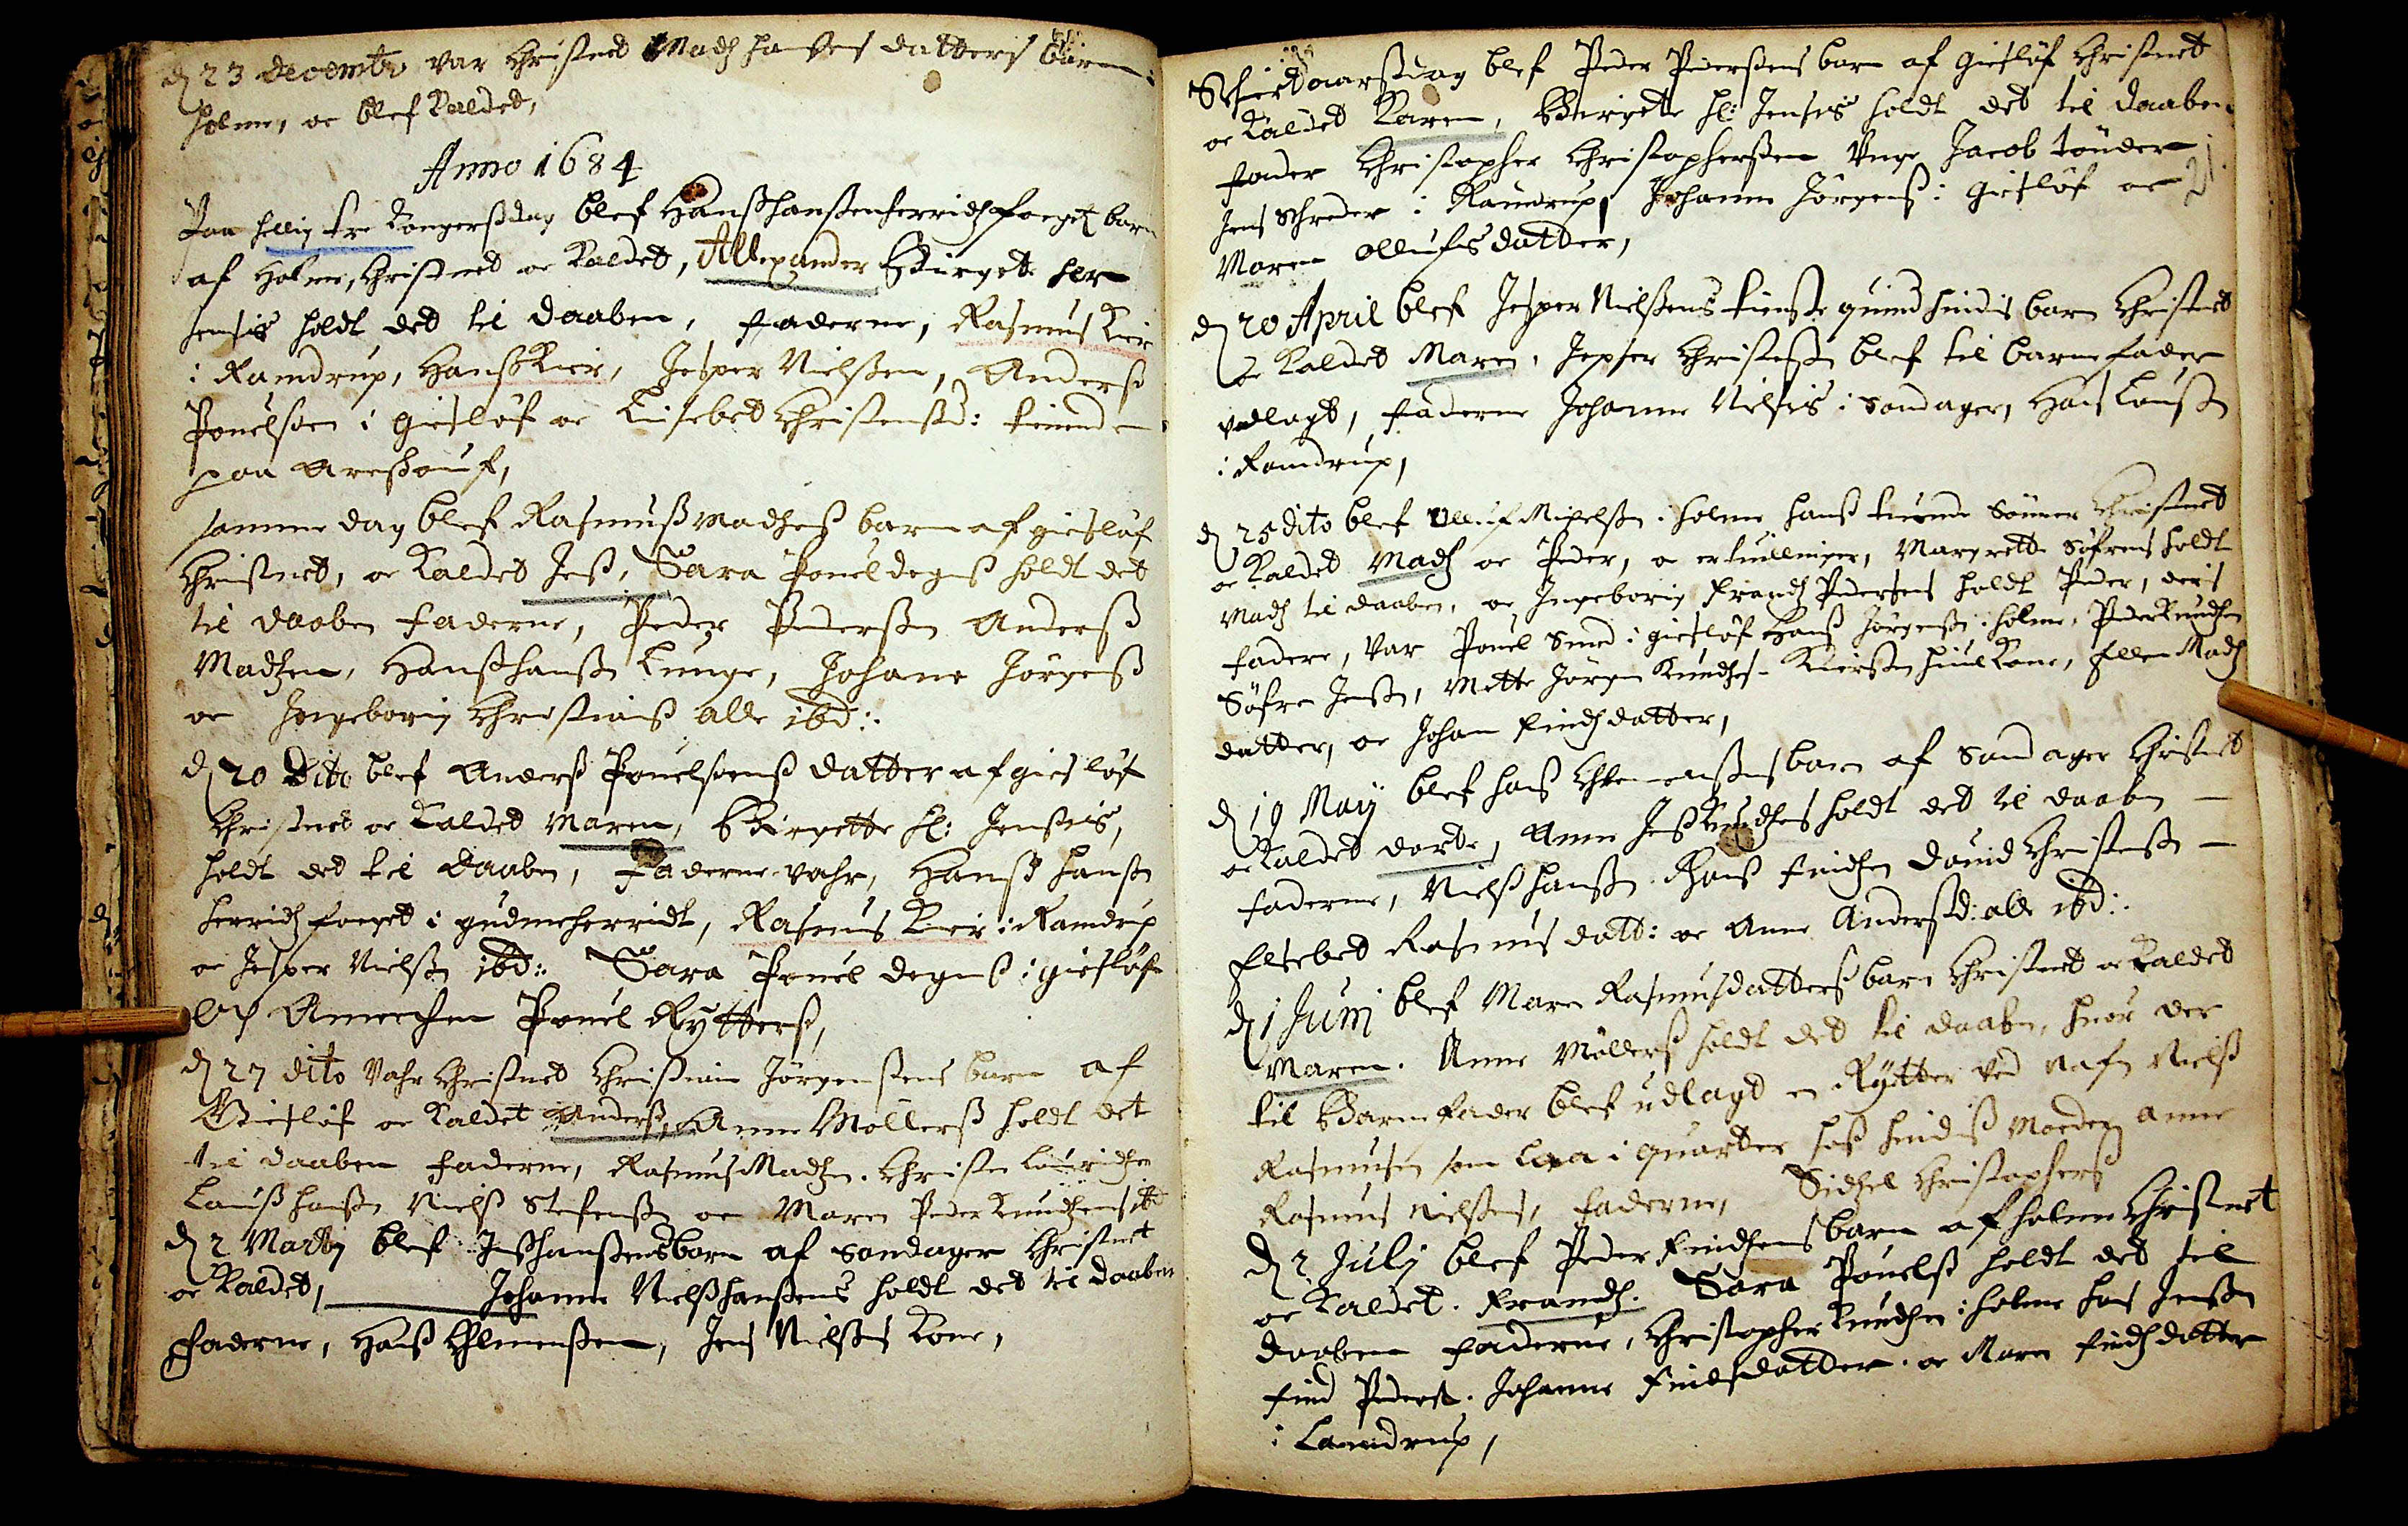d 23 Decembr var Christet Madtz Hansens datters barn i
Holme, oc blef kaldet,
Anno 1684
Paa hellig tre kongerssdag bleff Hanss Hanssen herridtzfoegets barn
af Holme Christened oc kaldet, Allexander Birgette hlr
Jensis holdt det til Daaben, Faderne; Rasmus Kier
i Ramdrup, Hans Kier, Jesper Nielssen, Anderss
Pouelsen i Gieløf oc Lisebet Christenssd: tiende
paa Areskouf,.
Samme dag blef Rasmuss Madtzens børn af giesløf
Christnet, oc kaldet Jens, Sara Pouel degnss holdt det
til daaben Faderne, Peder Pederssen Anderss
Madtzen, Hans Hanss Lunge, Johane Jørgenss
oc Ingeborig Christenss alle ibd:.
d 20 Dito blef Anderss Pouelsenss Datter af giesløf
Christnet oc kaldet Maren, Birrette Hl: Jenssis
holdt det til Daaben, Faderne vahr, Hanss Hanss
Herridtzfoeget i Gudmeherridt, Rasmus Kier i Ramdrup
oc Jesper Nielss ibd:. Sara Pouel Degns i giesløf
Och Anneche Pouel Rytterss,
d 27 dito Vahr Christian Jørgenssens barn af
Giesløf oc kaldet Anderss, Anne Møllerss holdt det
til daaben. Faderne, Rasmus Madtzen. Christen Lauridtzen
Laus Hanssen Nielss Stefenssen oc Maren Peder Knudtzens ibd
d 2 Martij blef Jens hansens barn af Sandager christnet
oc kaldet
Johanne Nielss Hansens holdt det tel Daaben
Faderne, Hans Chlemenssen, Jens Nielssis Kone,
21
Schirtaarsdag blef Peder Pederssens barn af Giesløf Christenet
kaldet Karen, Birgette Hl: Jensis holdt det til daaben
Fader Christopher Christopherssen vnge Jacob Tønder
Jens Schreder i Ramdrup, Johanen Jørgenss i Giesløf oc
Maren Ollufsdatter,
d 20 April blef Jeper Nielssens tienste quind hendis barn Christnet
oc kaldet Maren, Jepser Christesen blef til barnefader
vdlagt, Faderne Johanne Nielsis i Sandager, Hans Lauss
i Ramdrup
d 25 dito blef Olluf Michelsen i holme Hans tiunde Sønner Christenet
e kaldet Madtz oc Peder, oc er tuillinger, Margrette Søfrens holdt
Mads til daaben, oc Ingeborig Frandtz Pedersens holdt Peder, deris
Fadere, var Pouel Smed i Giesløf Hanss Jørgenssen i holme, Peder Knudtzen
Søfren Jenssen, Mette Jørgen Knudtzens Kiersten Hiul Kone, Ellen Madtz
datter, oc Johane Findtzdatter,
d 19 Maij blef Hanss Christenssens barn af Sandager Christenet
oc kaldet dorte, Anne Jens Knudtzens holdt det til Daaben
Faderne, Nielss Hanssen Hanss Findtzen Dauid Christenssen
Elsebet Rasmusdatt: oc Anne Anderssd: alde ibd:.
d 1 Junij blef Maren Rasmusdatters barn Christnet oc kaldet
Maren. Anne Møllerss holdt det til daaben, hvor der
til Barne fader blef udlagt en Rytter ved Nafn Nielss
Rasmusen som laa i Quarter hoss hindis Moeder Anne
Rasmus Nielsens, Faderne, Sidtzel Christopherss
d 2 Julij blef Peder Findtzens barn af holme Christenet
oc kaldet. Frandtz. Sara Pouels holdt det til
daaben Faderene, Christopher Knudtzen i holme hans Jenssen
find Peders. Johanne Findsdatter oc Maren Findsdatter
i Lamdrup,
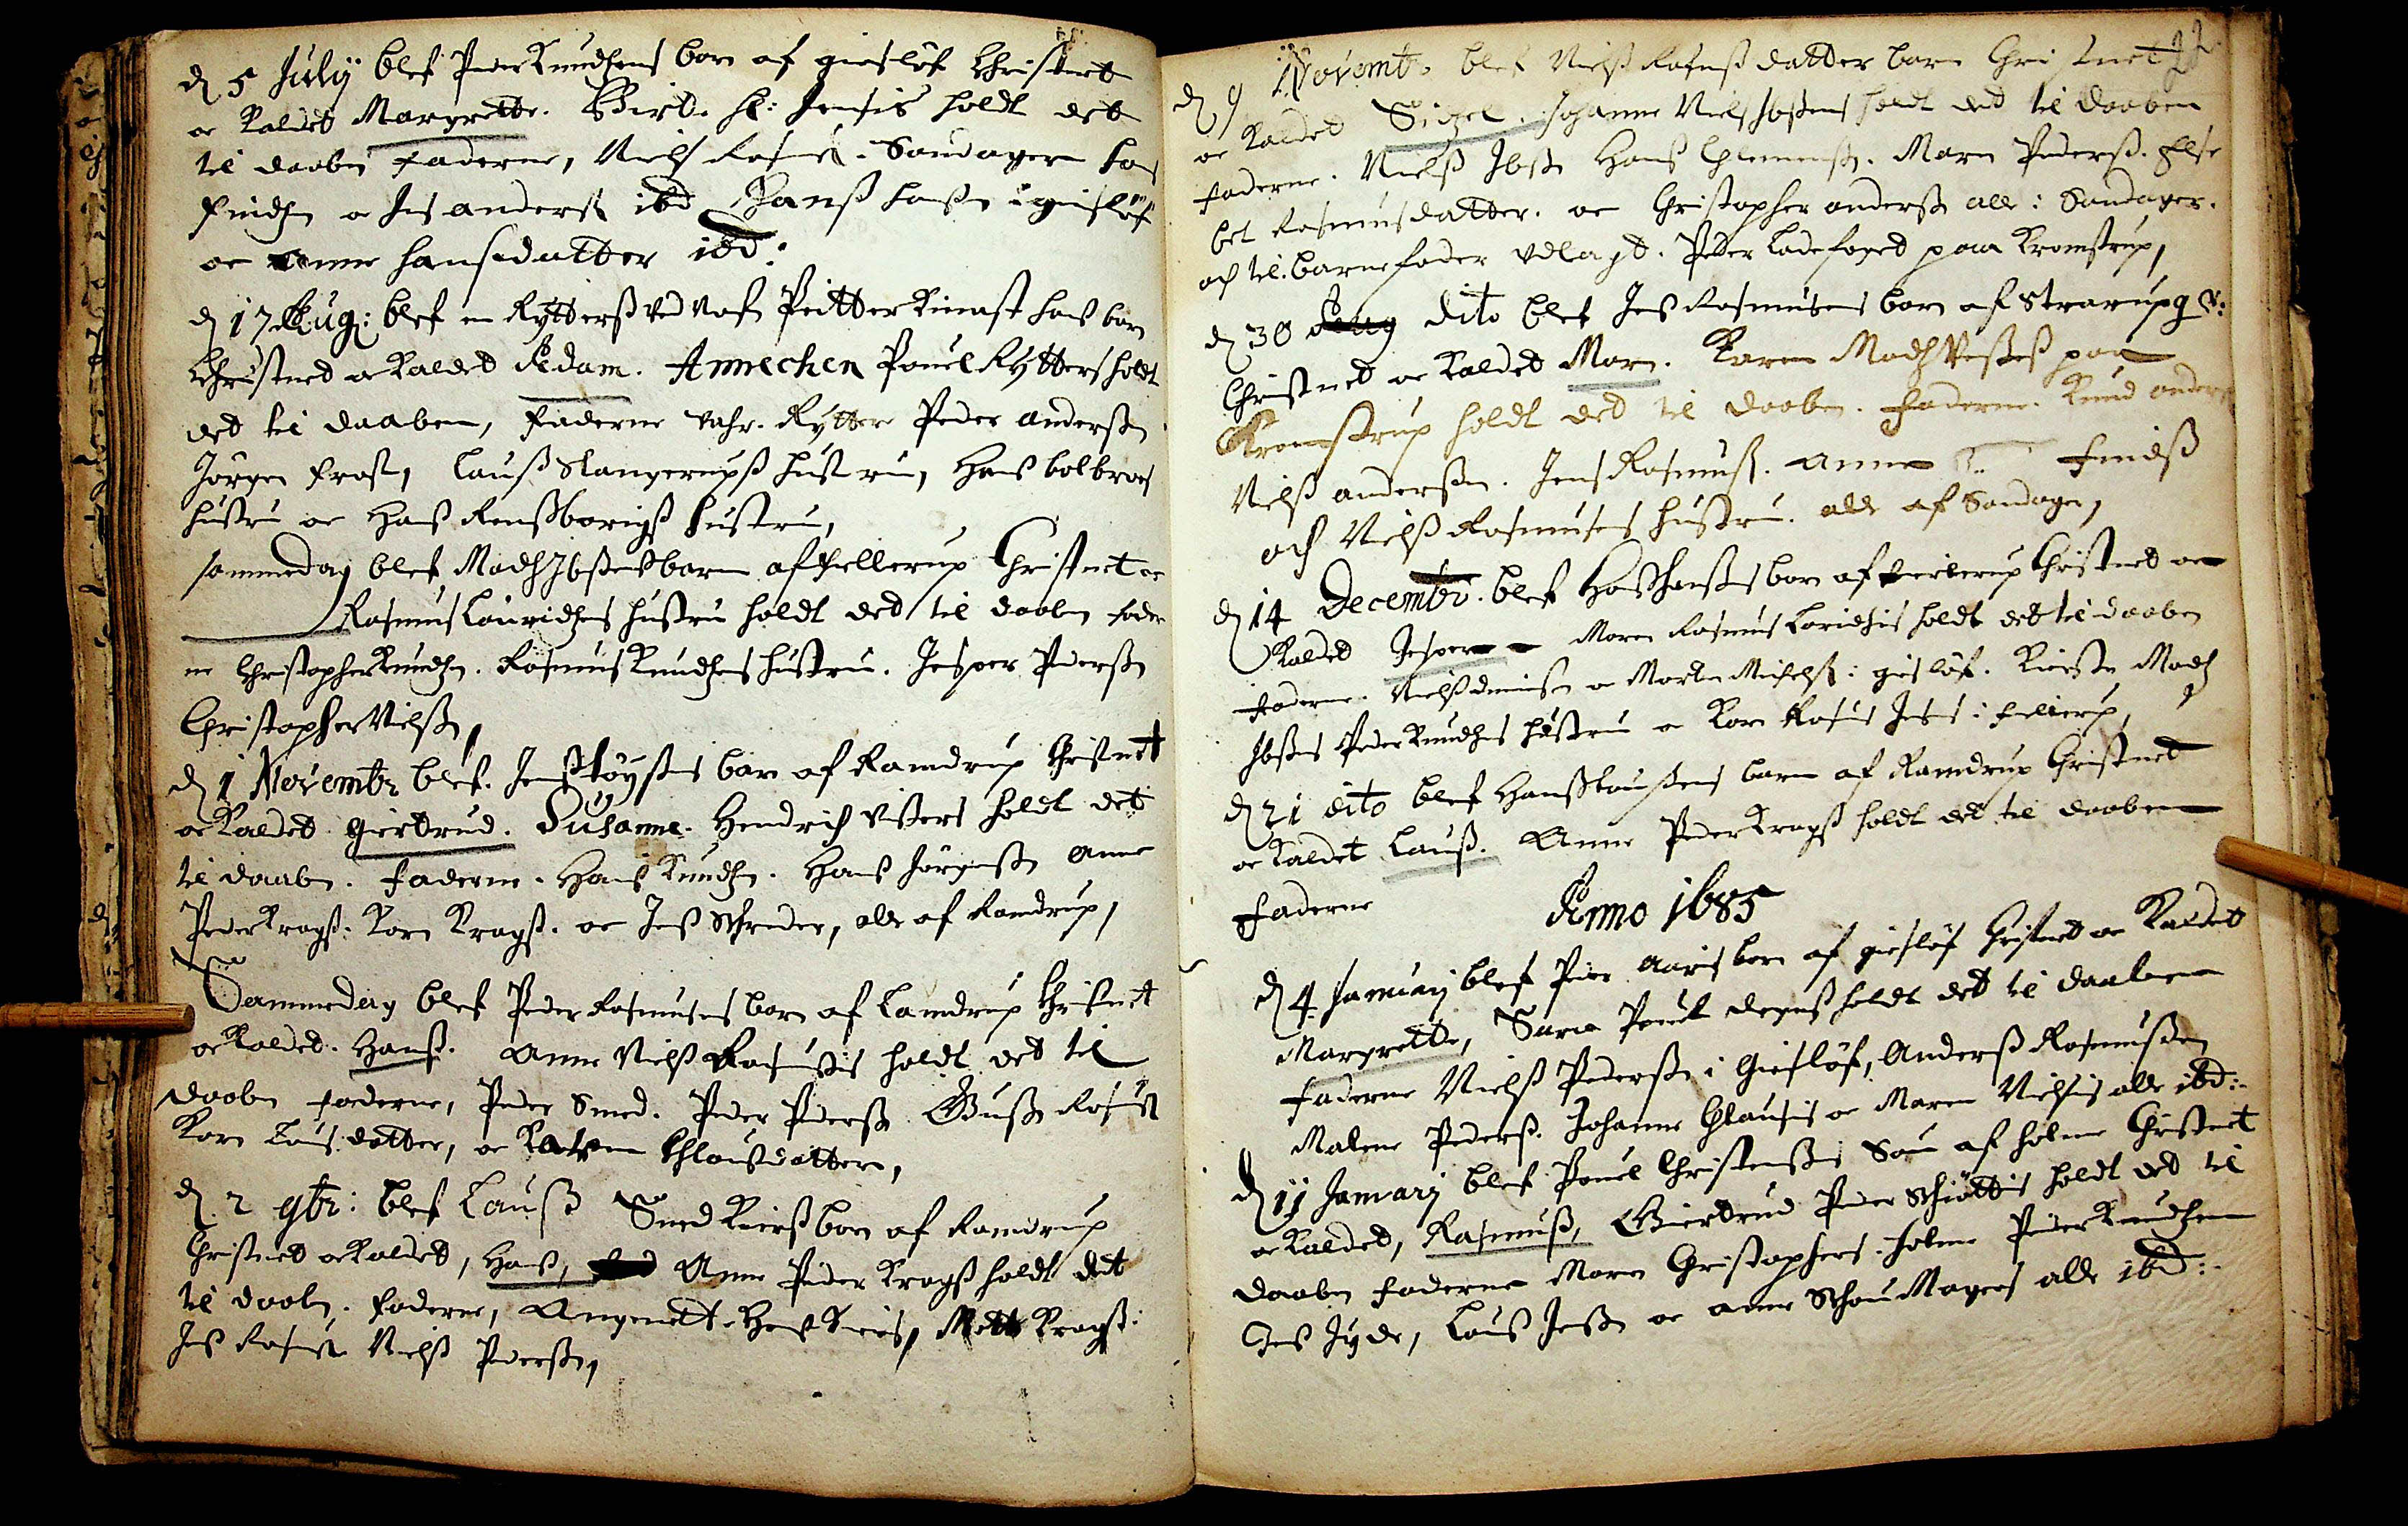d 5 Julij blef Peder Knudtzens barn af giesløf Christnet
oc kaldet Margrette. Birte Hl: Jensis holdt dett
til daaben Faderne, Niels Ramus i Sandager hos
Findtzen oc Jens Anders ibd. Hanss Hanssen i giesløf
oc Anne Hansdatter ibd.:
d 17 Aug: blef en Rytterss ved Nafn Peitter Kienest hans barn
Christned oc kaldet Adam. Annechen Pouel Rytters holdt
det til daaben, Faderne vahr Rytter Peder Anderssen
Jørgen Frost, Lauss Slangerupss hustru, Hanss bolbroes
Hustru oc Hanss Renssborigss hustru,
sammedag blef Madtz Ibssenss barn af fiellerup Christenet oc
Rasmus Lauridtzens hustru holdt det til daaben Føder
ne Christopher Knudtzen. Rasmus Knudtzens hustru. Jesper Pederssen
Christopher Nielss
d 1 Novembr blef Jens tøystes barn af Ramdrup Christnet
oc kaldet Giertrud. Susanne Hendrich Visters holdt det
til daaben. Faderne. Hanss Knudtzen. Hans Jørgenssen Anne
Peder Kragss: Karn Kragss. oc Jenss Schreder, alle af Landerup,
Sammedag blef Peder Rasmusens barn aff Lamdrup Christnet
oc kaldet. Hanss. Anne Nielss Rasmussis holdt det til
daaben faderne, Peder Smed: Peder Pederss Guste Rasmus
Karn Lausdatter, oc Katrine Chlausdatter,
d 2 9br: blef Lauss Smed Kierss born aff Ramdrup
Christnet oc kaldet, Hanss, vad Anne Peder Kragss holdt det
til daaben. Faderne Angenette Hans kiers,, Mette Kragss
Jens Rasmus Nielss Pederssen
22
d 9 Novemb. blef Niess Lafnss datter barn Christnet
kaldet Siczel, Joh anne Niels ossens hoidt det til Daaben
Faderne: Nielss Jbssn Hans Chlemensen. Maren Peders Else
bes Rosmnsdatter: oc Christopher Anderss alle i Søndager
och til barneføder vdlagt: Pdder Ladeloged paa Broenstrup
d 38 Datag dito blef Jes Resmusens barn aff Strarup g o
Christnet oc kaldet Mar. Karen Mads Veses paa
Bromstrup holdt ded til daaben. Faderne Knend Anders
Niels Andersøn: Jens Røsns. Anne h Fiids
och Niels Rosmusen hustru. ælde af Søndagen,
d 14 Decembr: blef Hos hanss born aff Berterup Christnet om
kaldet Jesper. Maren Rasmus Boridsis holdt det til daaben
Faderne Nielss derisen oc Morten Michels i grsløff. Kierste Mods
fesse Peder Knudses Fustru oc Kore Koss Jess i Fellerp
d 21 Oito blef Hans kousens barn af Ramdrup Christnet
oc kaldet Haus. Anne Peder Trups holdt det til daaben
Faderne
Anno 1683
Jtsemmysbef Pen arsen af rcheft hestder Latd.
Margates Søarn fent Geref lt Nlisdatter.
Faderne Niels Pedersen i Gresløf, Anders Rosemusens
Matene Peders Johanne Clausis oc Maren Nielsis alde ibd:
d 17 Januarij bleff Pouel Christensens Sørn af holme Christenet
o kaded, Rasrensen Giertrud Peder shulders føldt og til
daaben Faderne Maren Christophers holme Peder Knudzen
Jes Jyde, Las Jess, oc Anter Scheis Mogers att ibd.